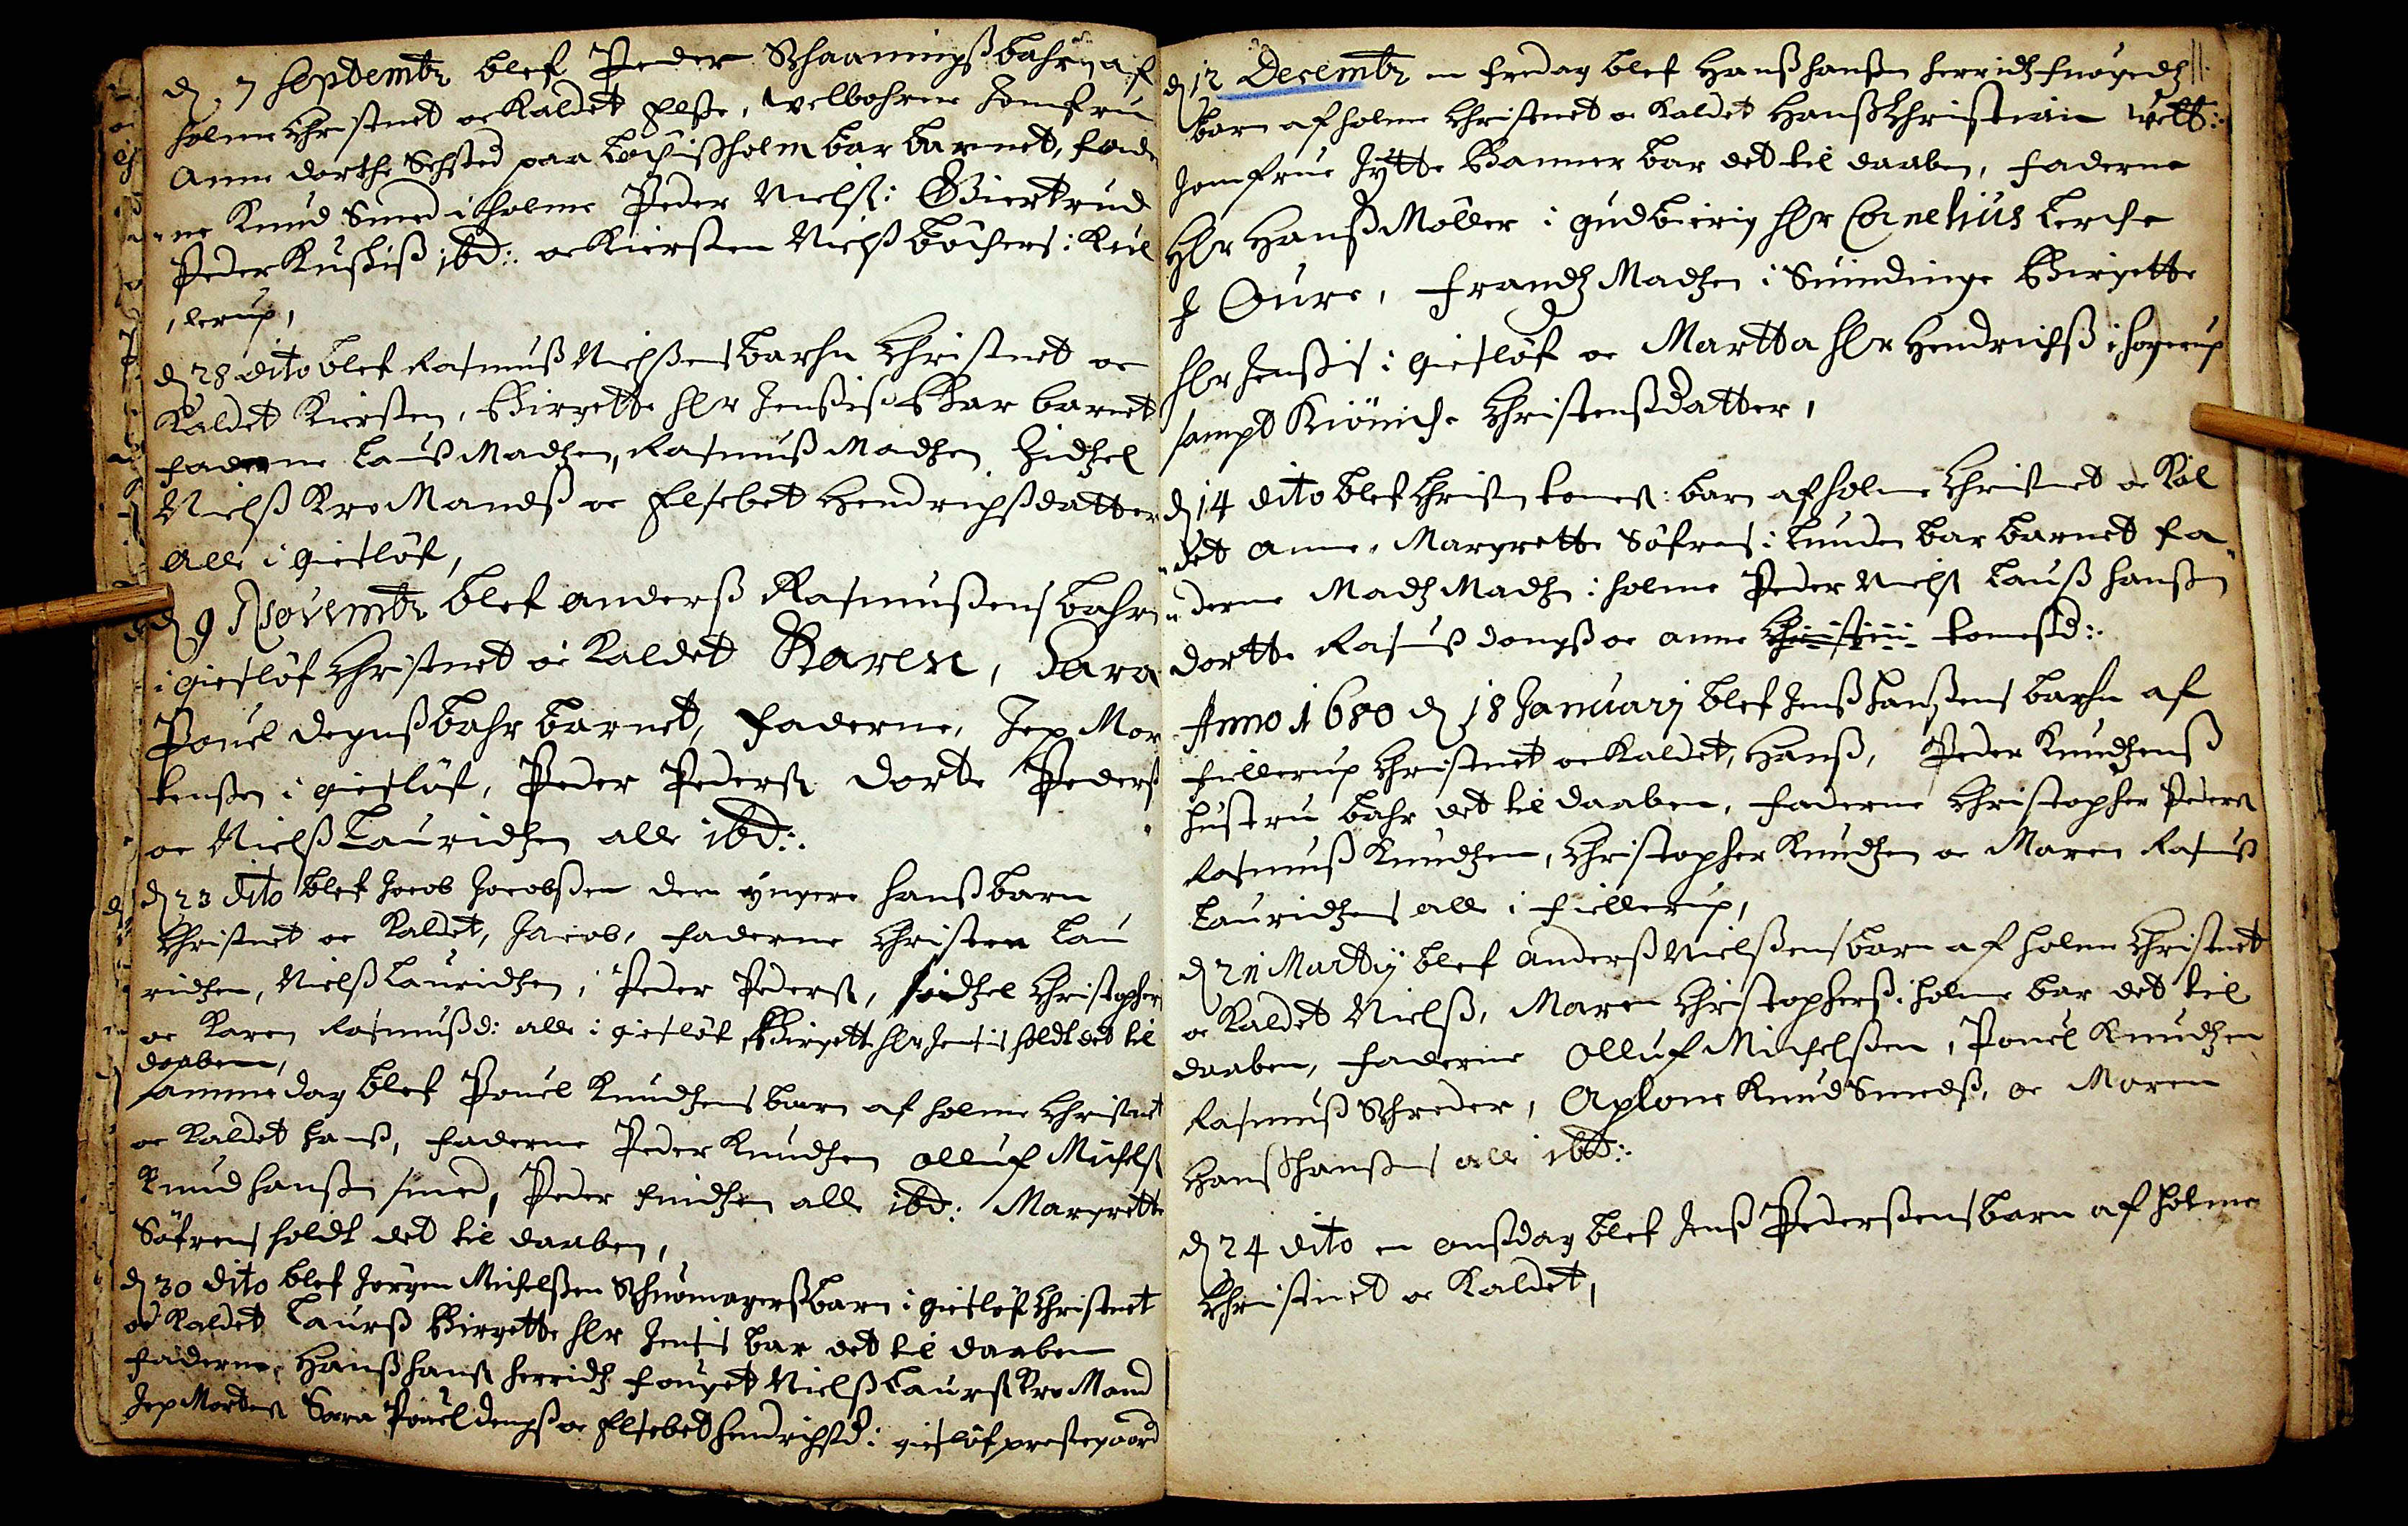d. 3 Sepdembr blef Peder Schaaningss bahrn af
Holme Christnet oc kaldet Elsse, Velbohrne Jomfru
Anne Dorthe Sehsted paa Løchissholm bar barnet, fader
= ne Knud Smed i Holme Peder Nielss i Giertrud
Peder Kuskiss ibd:. oc Kiersten Niels Bøchsers i Kul
= lerup.
D 28 dito blef Rasmus  Nielssens barhn Christnet oc
kaldet Kiersten, Birgette hlr Jenssiss Bar barnet
Faderme Lauss Madtzen, Rasmuss Madtzen Sidtzel
Nielss Kro Mandss oc Elsebet Hendrichssdatter
Alle i Giesløf
d. 9 Novembr blef Anderss Rasmussens bahrn
i Giesløf Christenet oc kaldet Karen, Sara
Pouel Degns bahr barnet, Faderne, Jep Mor
tenssen i Giesløf, Peder Peders Dorte Peders
oc Nielss Lauridtzen alle ibd:.
d 23 dito blef Jacob Jacobssen den yngere hans barn
Christnet oc kaldet, Jacob, Faderne Christen Lau
ridtzen, Niels Lauridtzen, Peder Peders, sidzel Christopher
oc Karen Rasmussd: alle i Giesløf, Birgette hlr Jensis holdt det til
daaben
samme dag blef Pouel Knudtzens barn af Holme Christenet
oc kaldet Hanss, Faderne Peder Knudtzen Olluf Michels
Knud Hanssen smed, Peder Findtzen alle ibd: Margrette
Søfrens holdt det til daaben,
d 30 dito blef Jørgen Michelssen Schuomagerss barn i Gietløf Christenet
oc kaldet Laurss Birgette hlr Jensis bar det til daaben
Faderne Hanss Hans Herridtz fouget Nielss Laurss Kro Mand
Jep Mortens. Sara Pouel dengss oc Elfebet Hendrichsd i giesløf prestegaard
11.
d 12 Decembr en fredag blef Hanss Hansen Herridtzfuogedtz 
barn af Holme Christnet oc kaldet Hans Christian Vetb:.
Jemfrue Jytte Barmer bar det til daaben, Faderne
Hlr Hans Møller i Gudbierig hlr Cornelius Lerche
I Oure, Frandtz Madtzen i Suindinge Birgette
hlr Jenssis i Giesløf oc Martta hlr Hendrichss i
sampt Kiømche Christensdatter,
d 14 dito blef Christen tomess barn af Holme Christnet oc Kal
det Anne, Margrette Søfrens i Lunden bar barnet fa¬
= derne Madtz Madtz i holme Peder Niels Lauss Hanssen
Dortte Rasmusss Dongss oc Anne Christen tomessd:.
Anno 1680 d 18 Januarij blef Jenss Hanssens barhn af
fiellerup Christnet oc kaldet Hanss, Peder Knudtzenss
Hustrue bahr det til daaben, Faderne Christopher Peders
Rasmuss Knudtzen, Christopher Knudtzsen oc Maren Rasmuss
Lauridtzes alle i Fiellerup
d 21 Martij blef Anderss Nielssens barn af Holme Christnet
oc kaldet Nielss, Maren Christopherss i Holme bar det til
daaben, Faderne Olluf Michelssen, Pouel Kirdtzen
Rasmuss Schreder, Aplone Knud Smeds, oc Maren
Hanss Hanssen alle ibd:.
d 24 dito en onssdag blef Jenss Pederssens barn af Holme
Christenet oc kaldet,
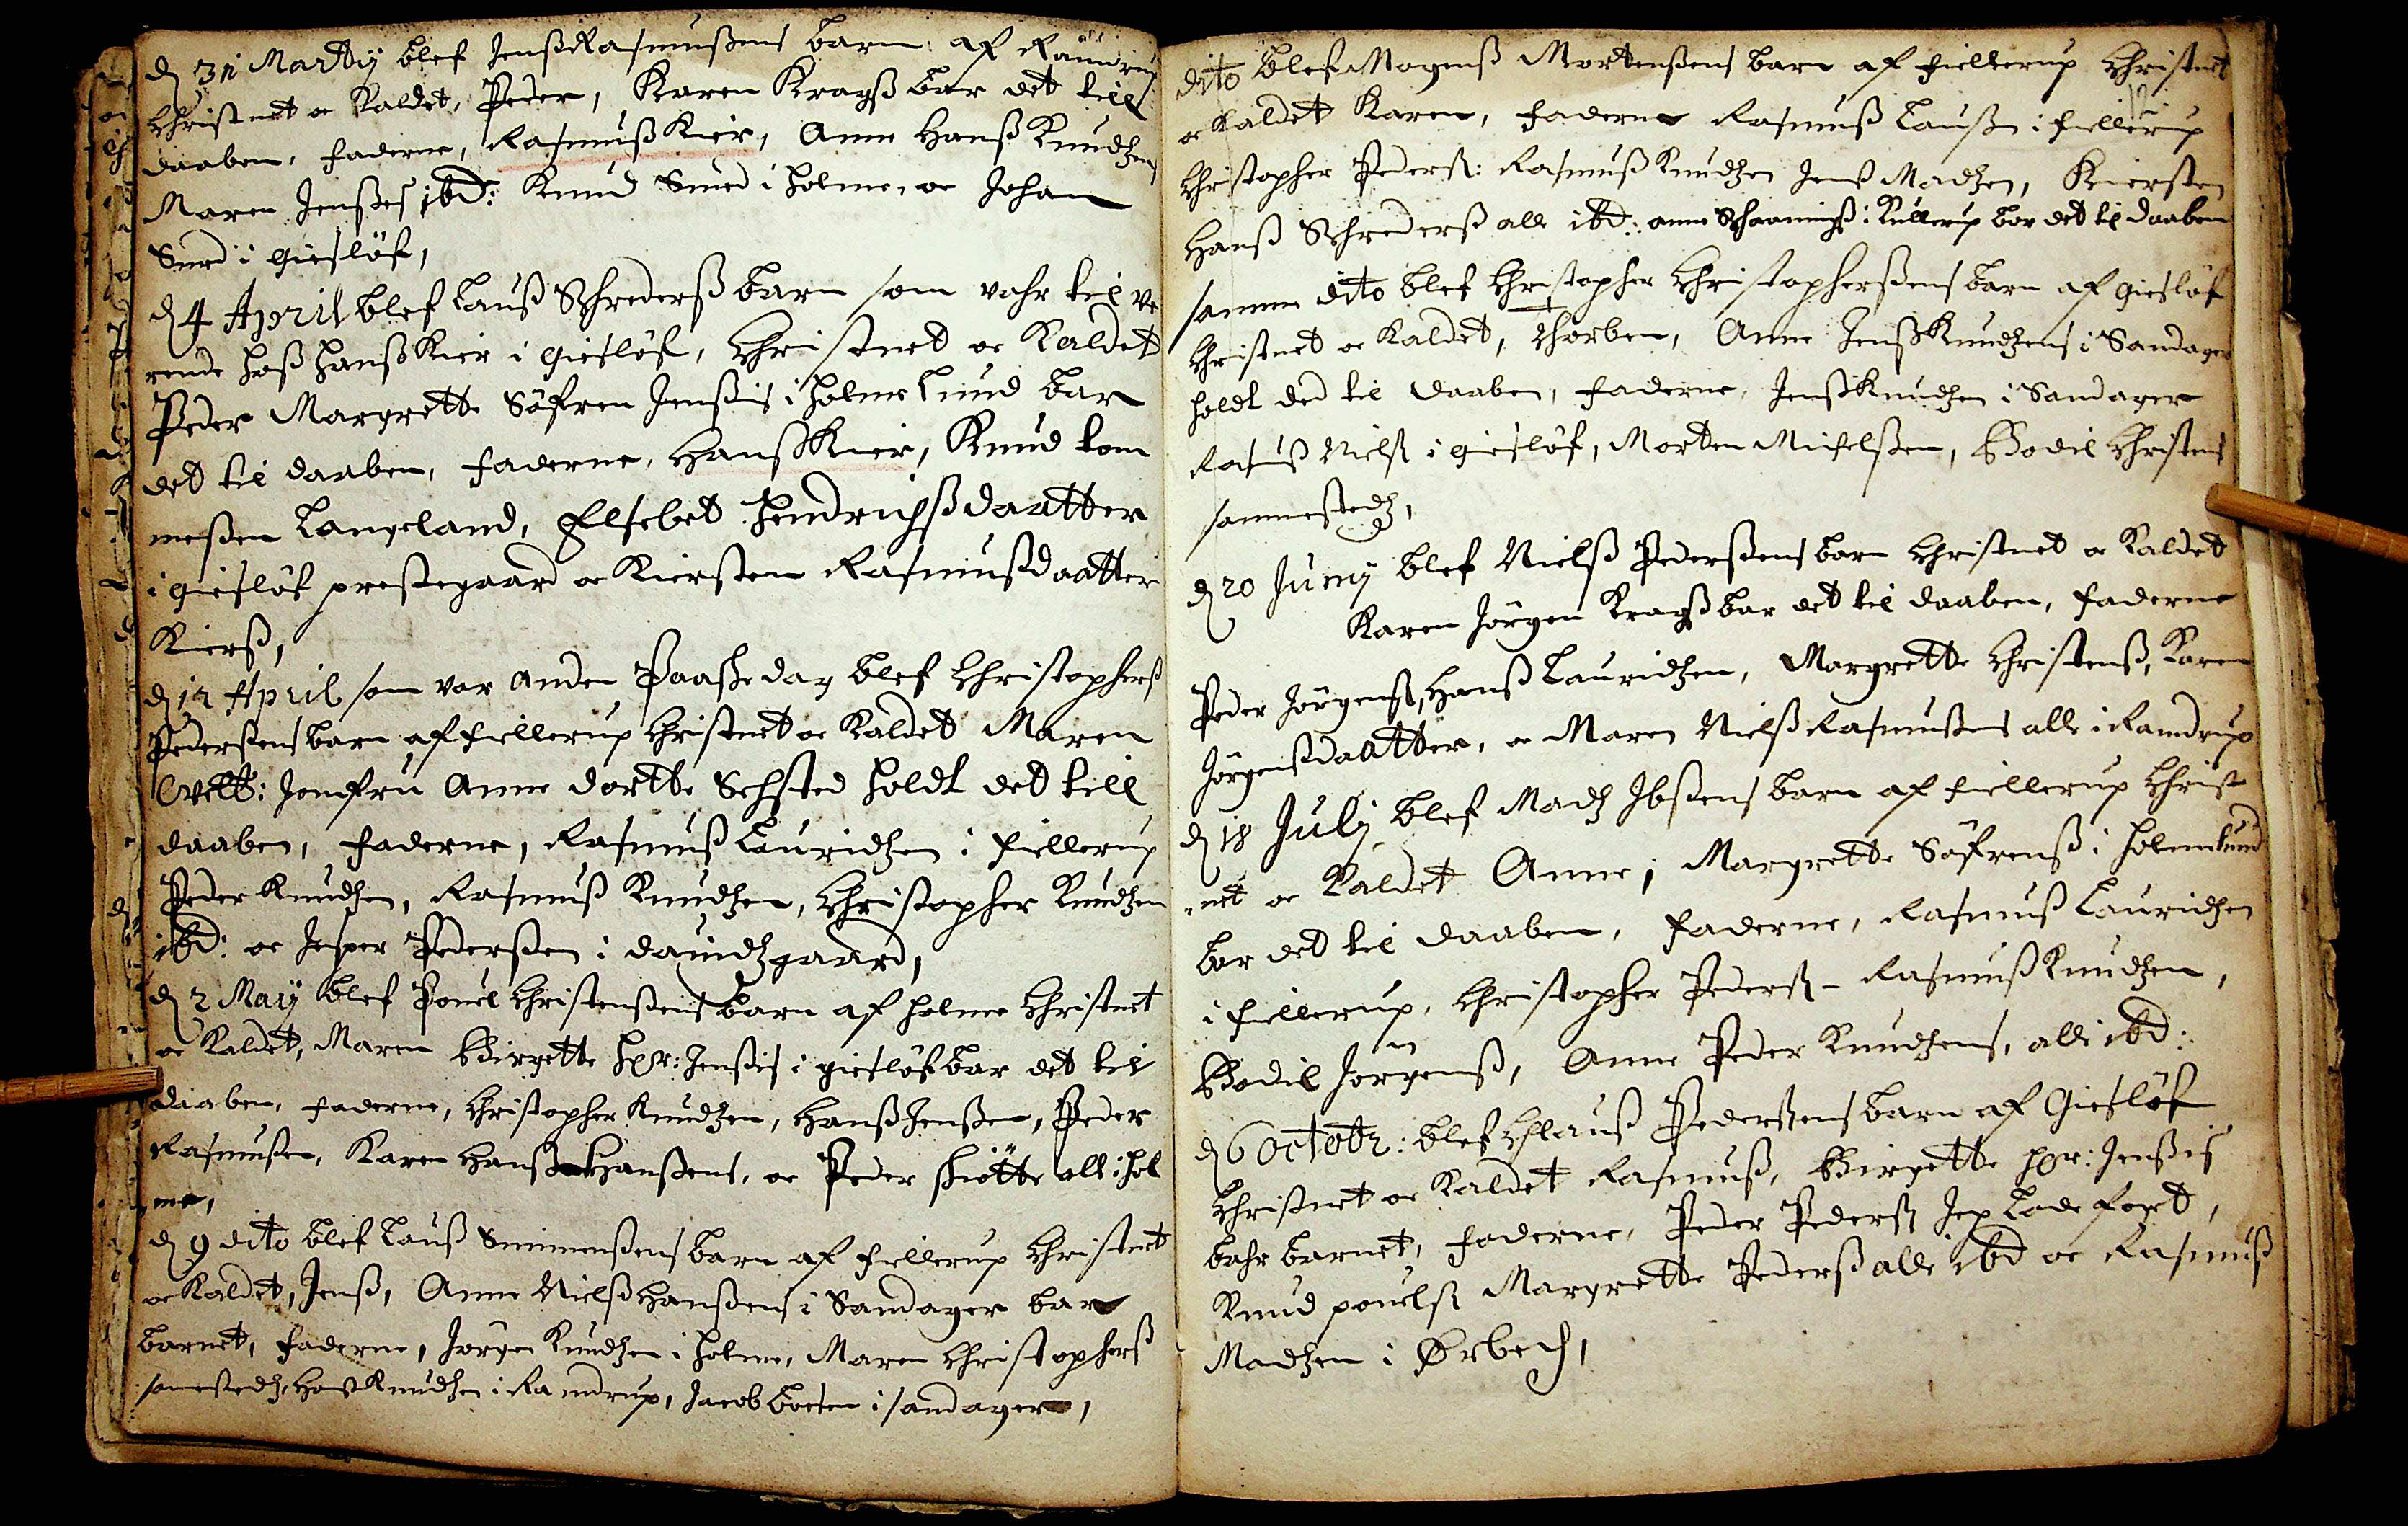d 31 Martij blef Jenss Rasmussens barn af Ramdrup
Christnet oc kaldet Peder, Karen Kragss bar det till
daaben. Faderne Rasmuss Kier, Anen Hanss Knudtzen
Maren Jensses ibd: Knud Smed i Holme, oc Johan
Smed i Giesløf,
d 4 April blef Lauss Schrederss barn som vahr til ve
rende Hanss Hansn Kier i Giesløf, Christned oc kaldet
Peder Margrette Søfren Jenssis i Holmelund bar
det til daaben, Faderne, Hanss Kier, Knud tom
messen Langeland, Elsebet Hendrichssdaatter
i Giesløf prestegaard oc Kiersten Rasmussdaatter
Kierst,
d 12 April som var anden Paaskedag blef Christopherss
Pederssens barn af Fiellerup Christnet oc kaldet Maren
Welb: Jomfru Anne Dortte Sehsted holdt det till
daaben, Faderne, Rasmus Lauridtzen i Fiellerup
Peder Knudtzen, Rasmuss Knudtzen, Christopher Knudtzen
ibd. oc Jesper Pederssen i Dauidtzgaard,
d 2 Maij blef Pouel Christenssens barn af holme Christnet
oc kaldet, Maren Birgette hlr: Jensis i giesløf bar det til
daaben, Faderne, Christopher Knudtzen, Hanss Jenssen, Peder
Rasmussen, Karen Hanss Hanssens, oc Peder Skiøtte all i Hol
me,
d 9 Sito blef Lauss Simmenssens barn af Fiellerup Christnet
oc kaldet Jenss, Anne Nielss Hanssen i Sandager bar
barnet, Faderne, Jørgen Knudtzen i Holme, Maren Christopherss
sammestedtz, Hanss Knudtzen i Ramdrup, Jacob boesen i sandagers,
12.
dito blef Mogenss Mortenssens barn af fiellerup Christenet
oc kaldet Karen, Faderne Rasmuss Lauss i fiellerup
Christopher Peders: Rasmuss Knudtzen Jens Madtzen, Kiersten
Hans Schrederss alle ibd:. anne Scha##gss i Kullerup bar det til Daaben
samme dito blef Christopher Christopherssens barn af Giesløf
Christenet oc kaldet, Thorben, Anne Jenss Knudtzens i Sandager
holdt ded til daaben, Faderne, Jenss Knudtzen i Sandagen
Rasmuss Niels i Giesløf, Morten Michelssen, Bodil Christens
sammestedtz,
d 20 Junij blef Niels Pederssens barn christnet oc kaldet
Karen Jørgen Kragss bar det til daaben, Faderne
Peder Jørgenss, Hans Lauridtzen, Margrette Christenss, Karen
Jørenssdaatter, oc Maren Nielss Rasmussens alle i Randrup
d 18 Julij blef Madtz Ibssens barn af Fiellerup Christ
= net oc kaldet Anne; Margrette Søfrenss i Holmelund
bar det til daaben, Faderne, Rasmus Lauridtzen
i Fiellerup, Christopher Peders - Rasmuss Knudtzen,
Bodil Jørgenss, Anne Peder Knudtzens, alle ibd:.
d 6 Octobr: blef Chlaus Pederssens barn af Giesløf
Christnet oc kaldet Rasmuss, Birgette hlr Jenssis
barr barnet, Faderne, Peder Peders, Jep Ladefoget,
Knud pouelss Margrette Pederss alte ibd oc Rasmuss
Madtzen i Ørbech,